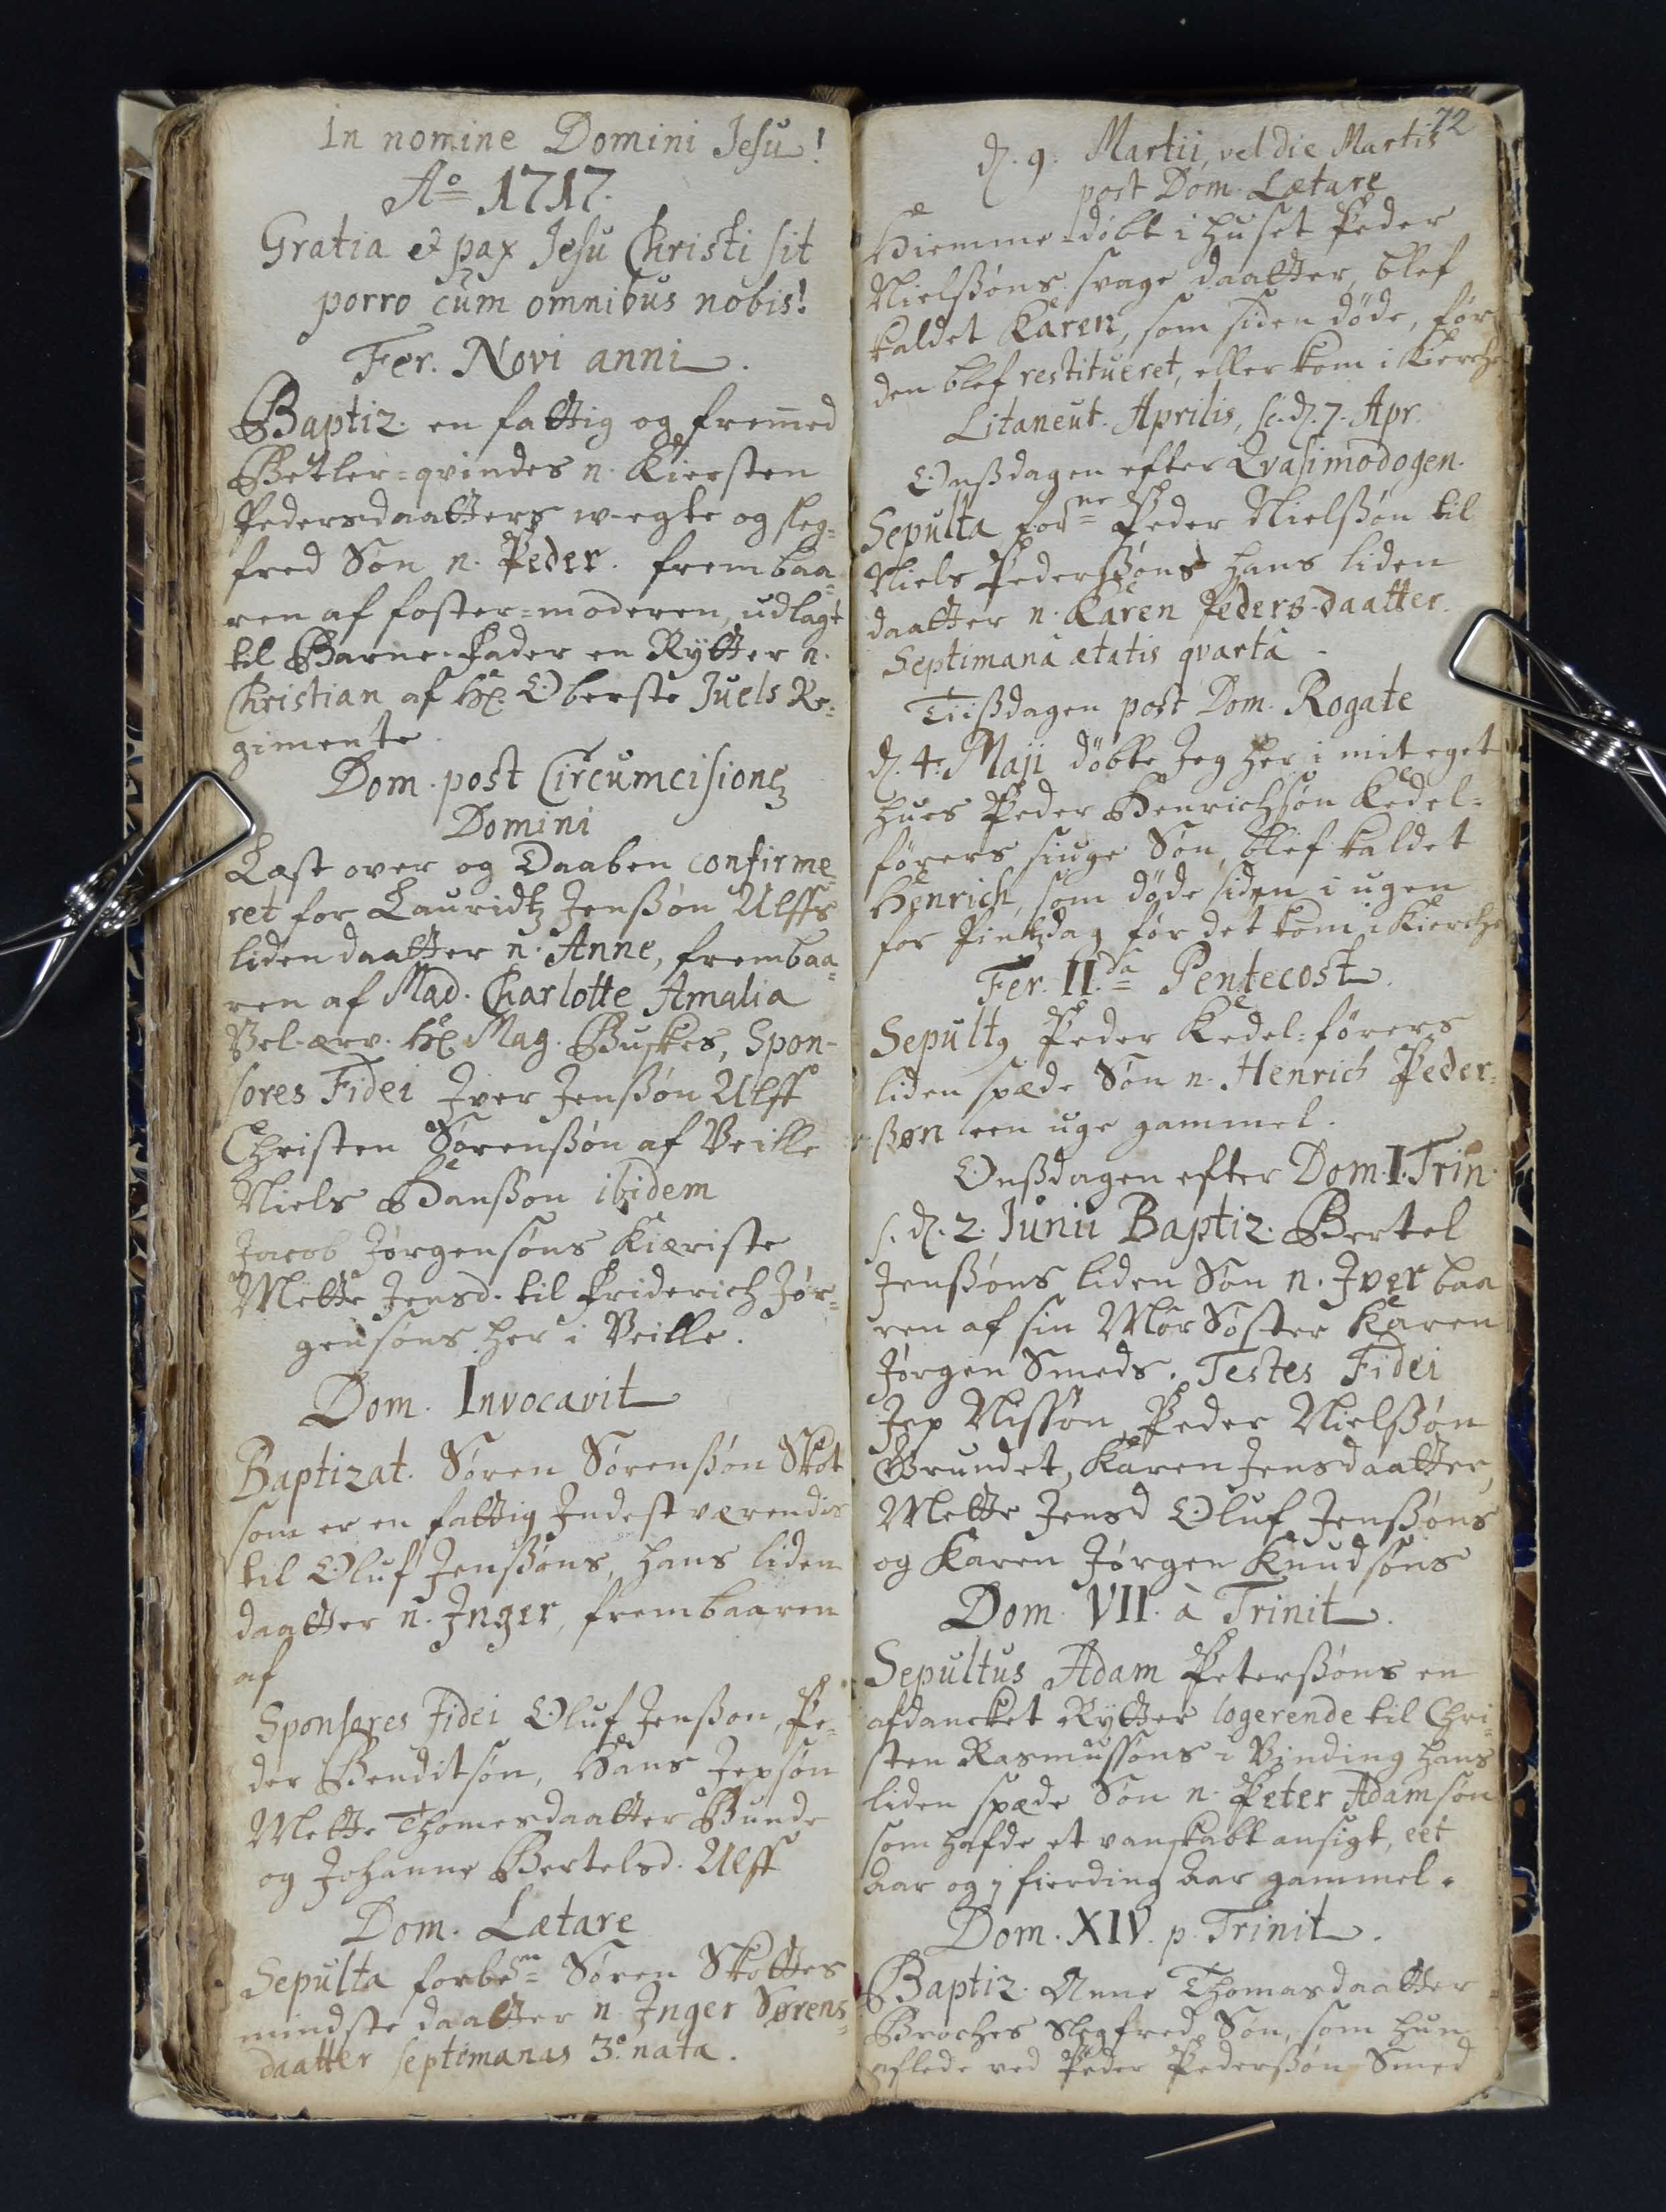In nomine Domini Jesu!
Ao 1717.
Gratia et pax Jesu Christi sit
porro cum omnibus nobis!
Fer. Novi anni
Baptiz. en fattig og fremmed
Betler=qvindes n. Kiersten
Pedersdaatters w-egte og sleg¬
fred Søn n. Peder. frembaa¬
ren af Foster=moderen, udlagt
til Barne-Fader en Rytter n.
Christian af Hl Oberste Juels Re¬
gimente
Dom. post Circumcisionez
Domini
Læst over og Daaben confirme¬
ret for Lauridtz Jenssøn Ulffs
liden daatter Anne, frembaa¬
ren af Mad. Charlotte Amalia
Vel-ærv. Hl Mag. Buskes, Spon¬
sores Fidei Iver Jenssøn Ulff
Christen Sørenssøn af Veille
Niels Hanssøn ibidem
Jacob Jørgensøns Kiæriste
Mette Jensd. til Friderich Jør¬
gensøns her i Veille
Dom. Invocavit
Baptizat. Søren Sørenssøn Skot
som er en fattig Indest værendis
til Oluf Jenssøns, hans liden
daatter n. Inger, frembaaren
af
Sponsores fidei Oluf Jenssøn, Pe¬
der Benditsøn, Hans Jepsøn
Mette Thomasdaatter Bunde
og Johanne Bertelsd. Ulff
Dom. Lætare
Sepulta forbe## Søren Skottes
mindste daatter n. Inger Sørens¬
daatter septimanas 3. nata.
72
d. 9. Martii, vel die Martis
post Dom. Lætare
Hiemmedøbt i Huset Peder
Nielssøns svage daatter, blef
kaldet Karen, som siden døde, før
den blef restitueret, eller kom i Kiercke
Litaneut. Aprilis, sc. d. 7. Apr.
Onssdagen efter Qvasimodogen.
Sepulta forne Peder Nielssøn til
Niels Pederssøns, hans liden
daatter n. Karen Pedersdaatter.
Septimana ætatis qvarta.
Tiissdagen post Dom. Rogate
d. 4. Maji. døbte Jeg her i mit eget
Hues Peder Henrichsøn Kedel¬
førers siuge Søn, blef kaldet
Henrich, som døde siden i ugen
for Pintzdag, før det kom i kierche
Fer. II. da Pentecost
Sepultq Peder Kedel=førers
liden spæde Søn n. Henrich Peder¬
ssøn een uge gammel
Onssdagen efter Dom. I. Trin.
s. d. 2 Junii. Baptiz. Bertel
Jenssøns liden Søn n. Iver, baa
ren af sin Mor Søster Karen
Jørgen Smeds. Testes Fidei
Jep Nissøn, Peder Nielssøn
Grundet, Karen Jensdaatter,
Mette Jensd Oluf Jenssøns
og Karen Jørgen Knudsøns
Dom. VII. a Trinit
Sepultus Adam Peterssøns en
afdancket Rytter logerende til Chri¬
sten Rasmussøns i Vinding hans
liden spæde Søn n. Peter Adamsøn
som hafde et vanskabt ansigt, eet
Aar og 1 fierding Aar gammel.
Dom. XIV. p. Trinit
Baptiz. Anne Thomasdaatter 
Broches slegfred Søn, som hun
aflede ved Peder Pederssøn Smed